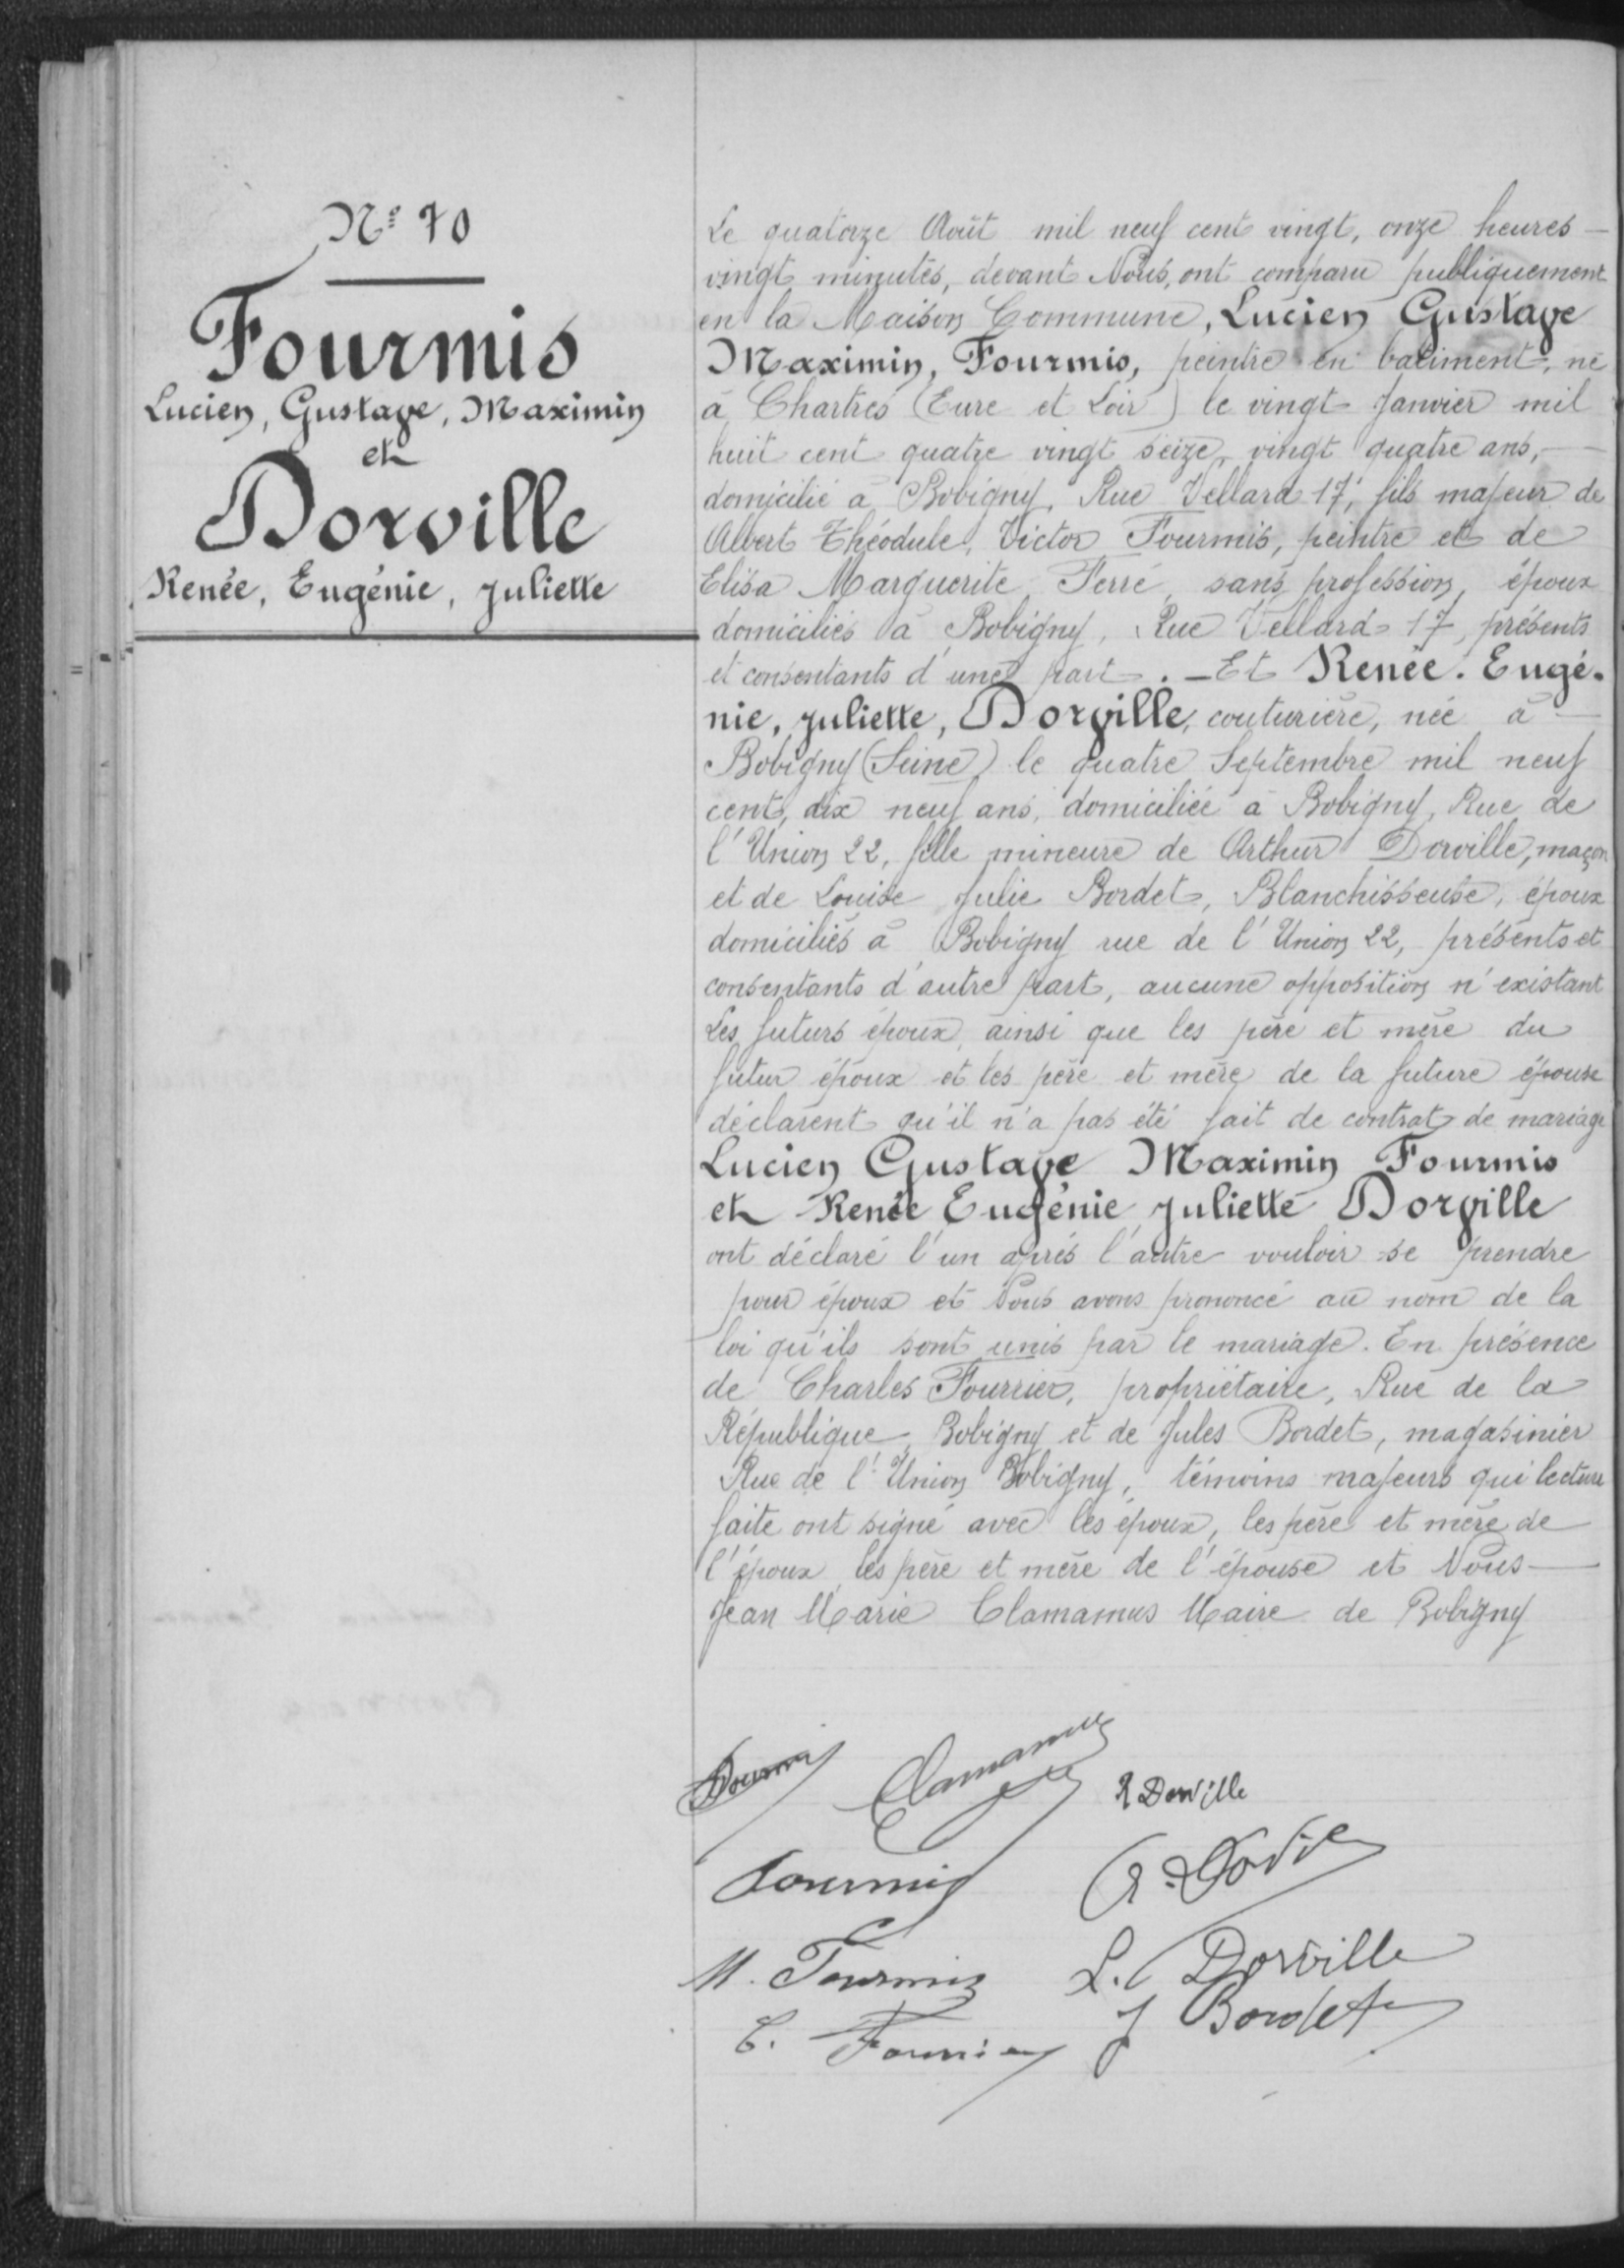N°:70
Fourmis
Lucien, Gustave, Maximin
et
Dorville
Renée, Eugénie, Juliette
Le quatorze août mil neuf cent vingt, onze heures
vingt minutes, devant Nous ont comparu publiquement
en la Maison Commune, Lucien Gustave
Maximin, Fourmis, peintre en batiment, né
à Chartres (Eure et Loir) le vingt Janvier mil
huit cent quatre vingt seize, vingt quatre ans,
domicilié à Bobigny, rue Vellard 17, fils majeur de
Albert Théodule, Victor Fourmis, peintre et et de
Elisa Merguerite Ferré, sans profession, époux
domiciliés à Bobigny, Rue Vellard 17, présents
et consentants d'une part .-Et Renée. Eugé-
nie, Juliette, Dorville couturière, née à
Bobigny (Seine) le quatre Septembre mil neuf
cents dix neuf ans, domiciliée à Bobigny, Rue de
l'Union 22, fille mineure de Arthur Dorville, maçon
et de Louise Julie Bordet, Blanchisseuse, époux
domiciliés à Bobigny rue de l'Union 22, présents et
consentants d'autre part, aucune opposition n'existant
Les futurs époux, ainsi que les père et mère du
futur époux et les père et mère de la future épouse
déclarent qu'il n'a pas été fait de contrat de mariage
Lucien Gustave Maximin Fourmis
et Renée Eugénie Juliette Dorville
ont déclaré l'un après l'autre vouloir se prendre
pour époux et Nous avons prononcé au nom de la
loi qu'ils sont unis par le mariage. En présence
de Charles Fourrier, propriétaire, Rue de la
République Bobigny et de Jules Bordet, magasinier
Rue de l'Union Bobigny, témoins majeur qui lecture
fatite ont signé avec les époux, les père et mère de
l'époux les père et mère de l'épouse et Nous
Jean Marie Clamamus Maire de Bobigny
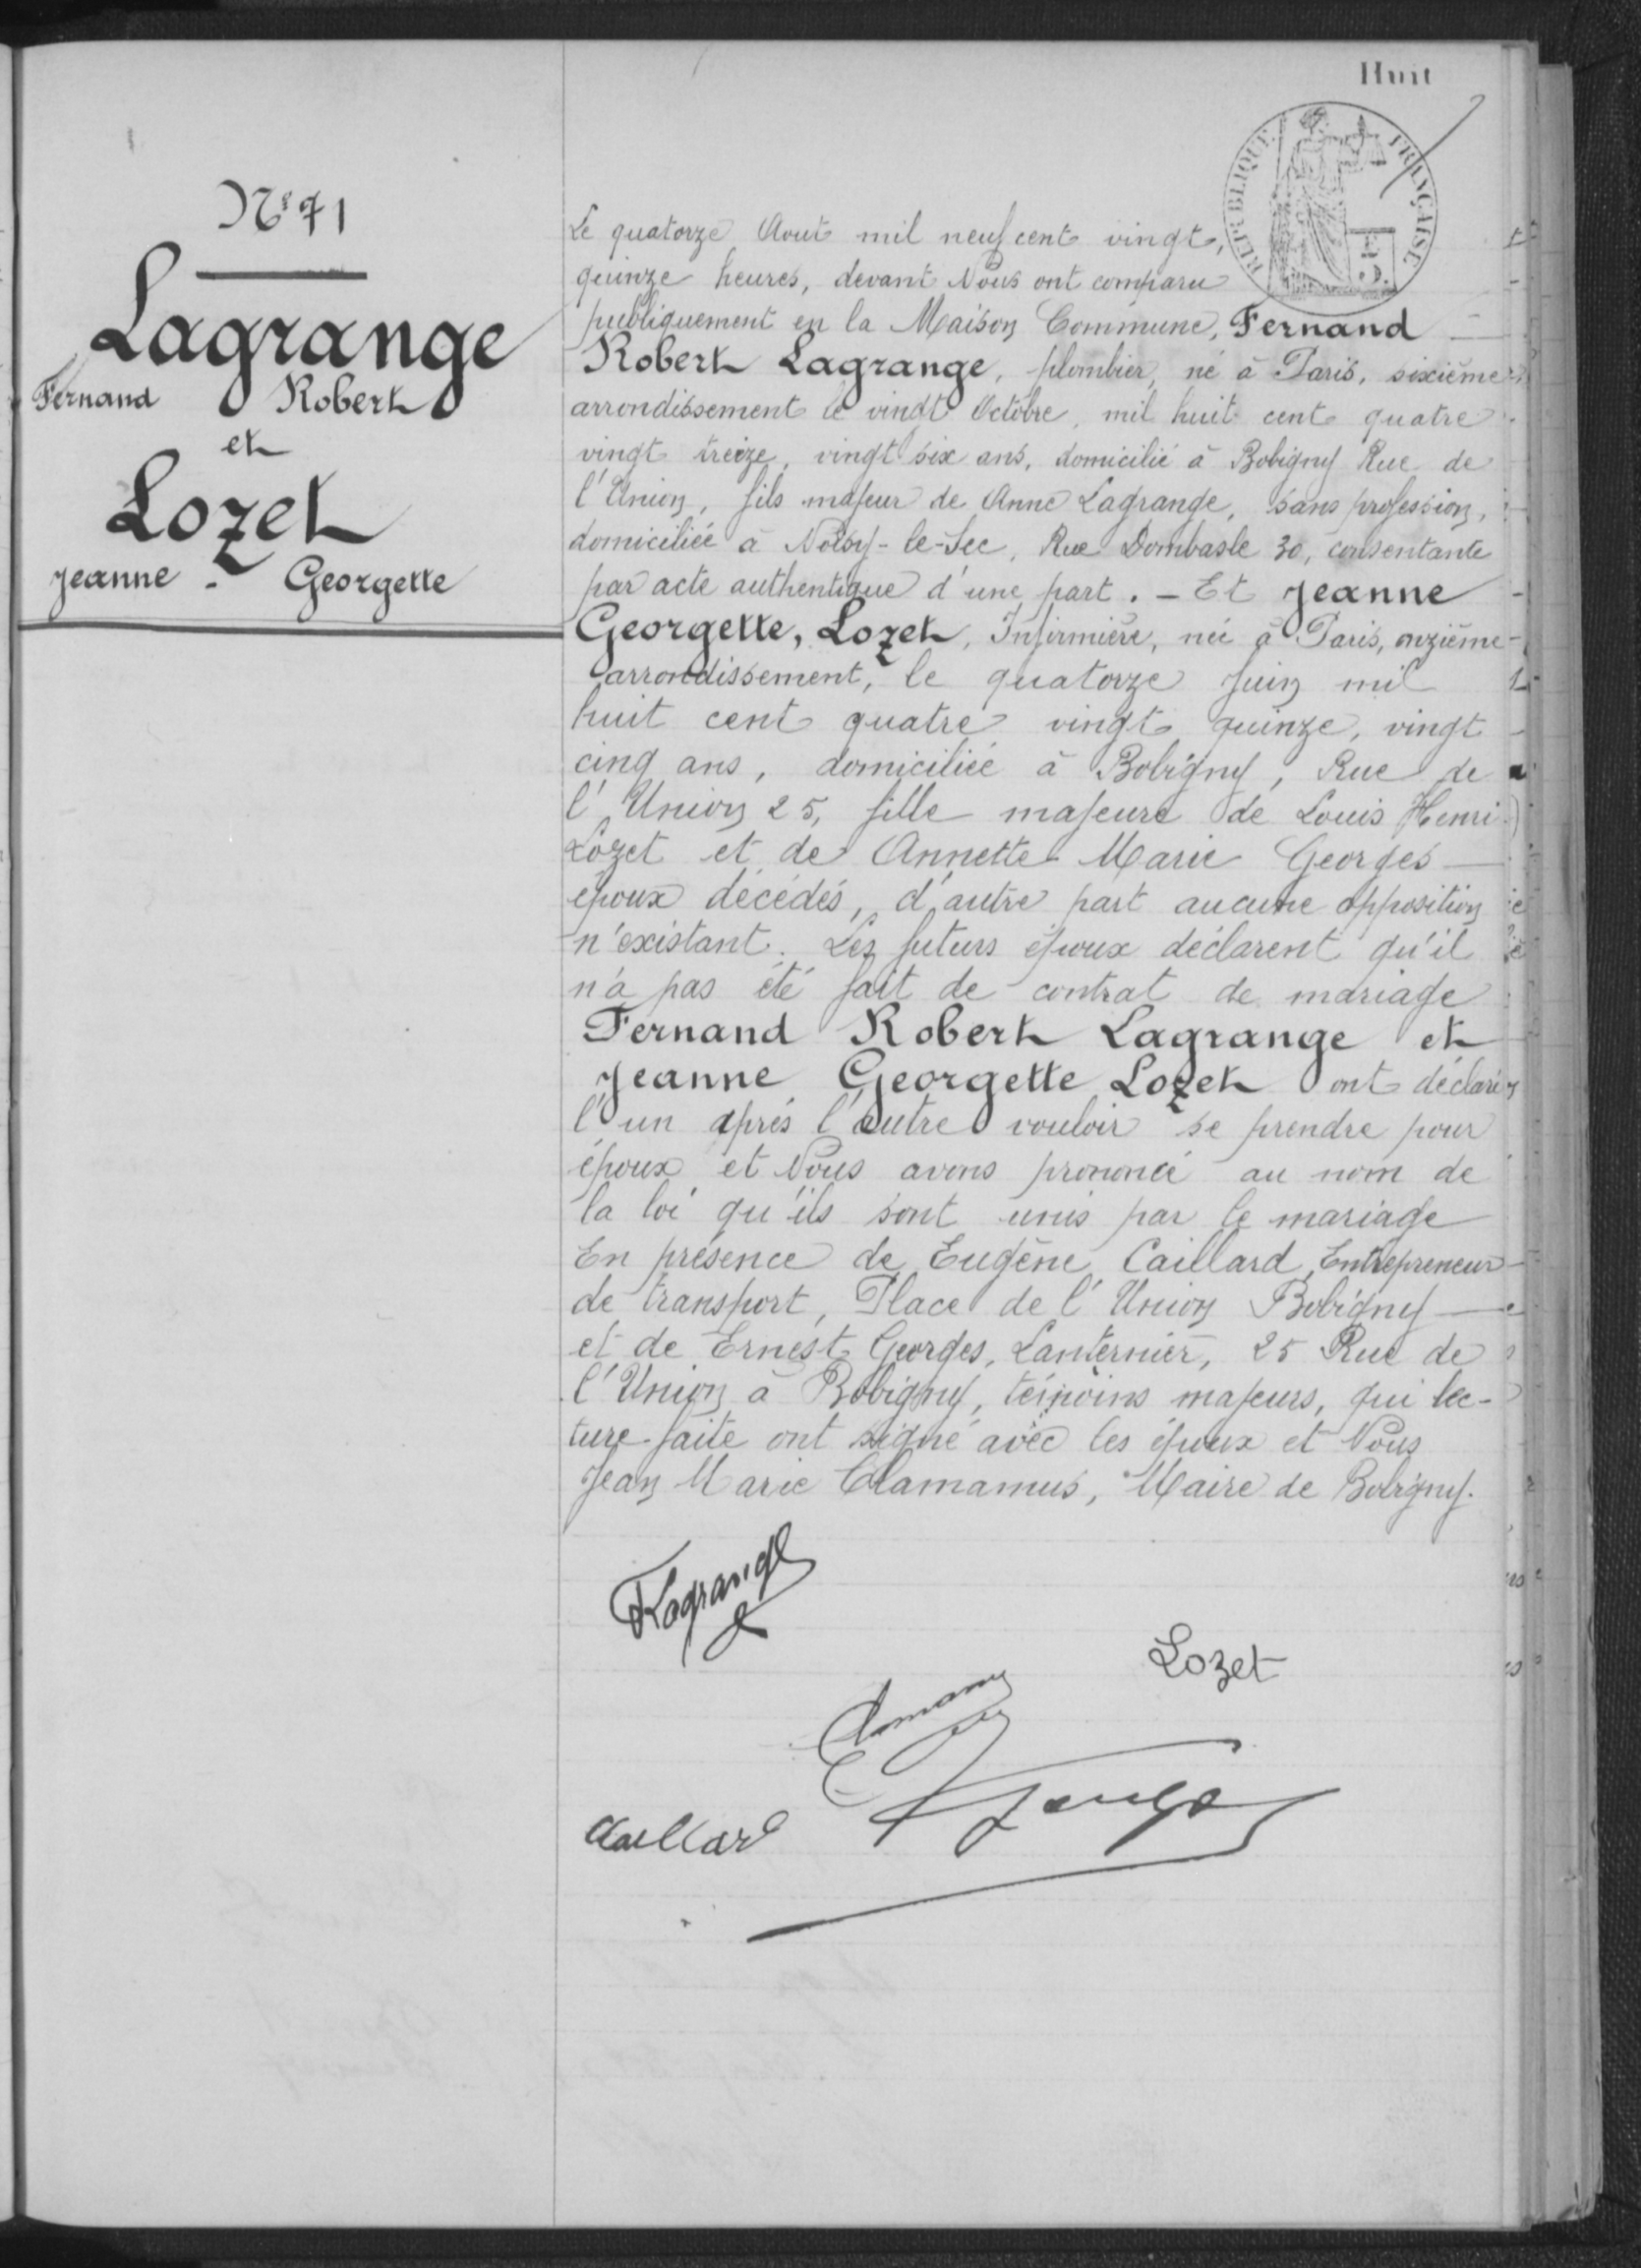N°71
Lagrange
Fernand Robert
et
Lozet
Jeanne-Georgette
Le quatorze Aout mil neuf cent vingt
quinze heures, devant Nous ont comparu
publiquement en la Maison Commune, Fernand
Robert Lagrange, plombier, né à Paris, sixième
arrondissement le vingt octobre, mil huit cent quatre
vingt treize, vingt six ans, domicilié à Bobigny Rue de
l'Union, fils majeur de Anne Lagrange, sans profession,
domiciliée à Noisy-le-Sex, Rue Dombasle 30, consentante
par acte authentique d'une part.-Et Jeanne
Georgette, Lozet, Infirmière, née à Paris, onzième
arrondissement, le quatorze Juin mil
huit cent quatre vingt quinze, vingt
cinq ans, domiciliée à Bobigny, Rue de
l'Union 25, fille majeure de Louis Henri
Lozet et de Annette Marie Georges
époux décédés, d'autre part aucune opposition
n'existant. Les futurs époux déclarent qu'il
n'a pas été fait de contrat de mariage
Fernad Robert Lagrange et
Jeanne Georgette Lozet ont déclaré
l'un après l'autre vouloir se prendre pour
époux et Nous avons prononcé au nom de
la loi qu'ils sont unis par le mariage
En présence de Eugène Caillard, Entrepreneur
de transport, Place de l'Union Bobigny
et de Ernest Georges Lanternier, 25 Rue de
l'Union à Bobigy témoins majeurs qui lec-
ture faite ont signé avec les époux et Nous
Jean Marie Clamamus, Maire de Bobigny.